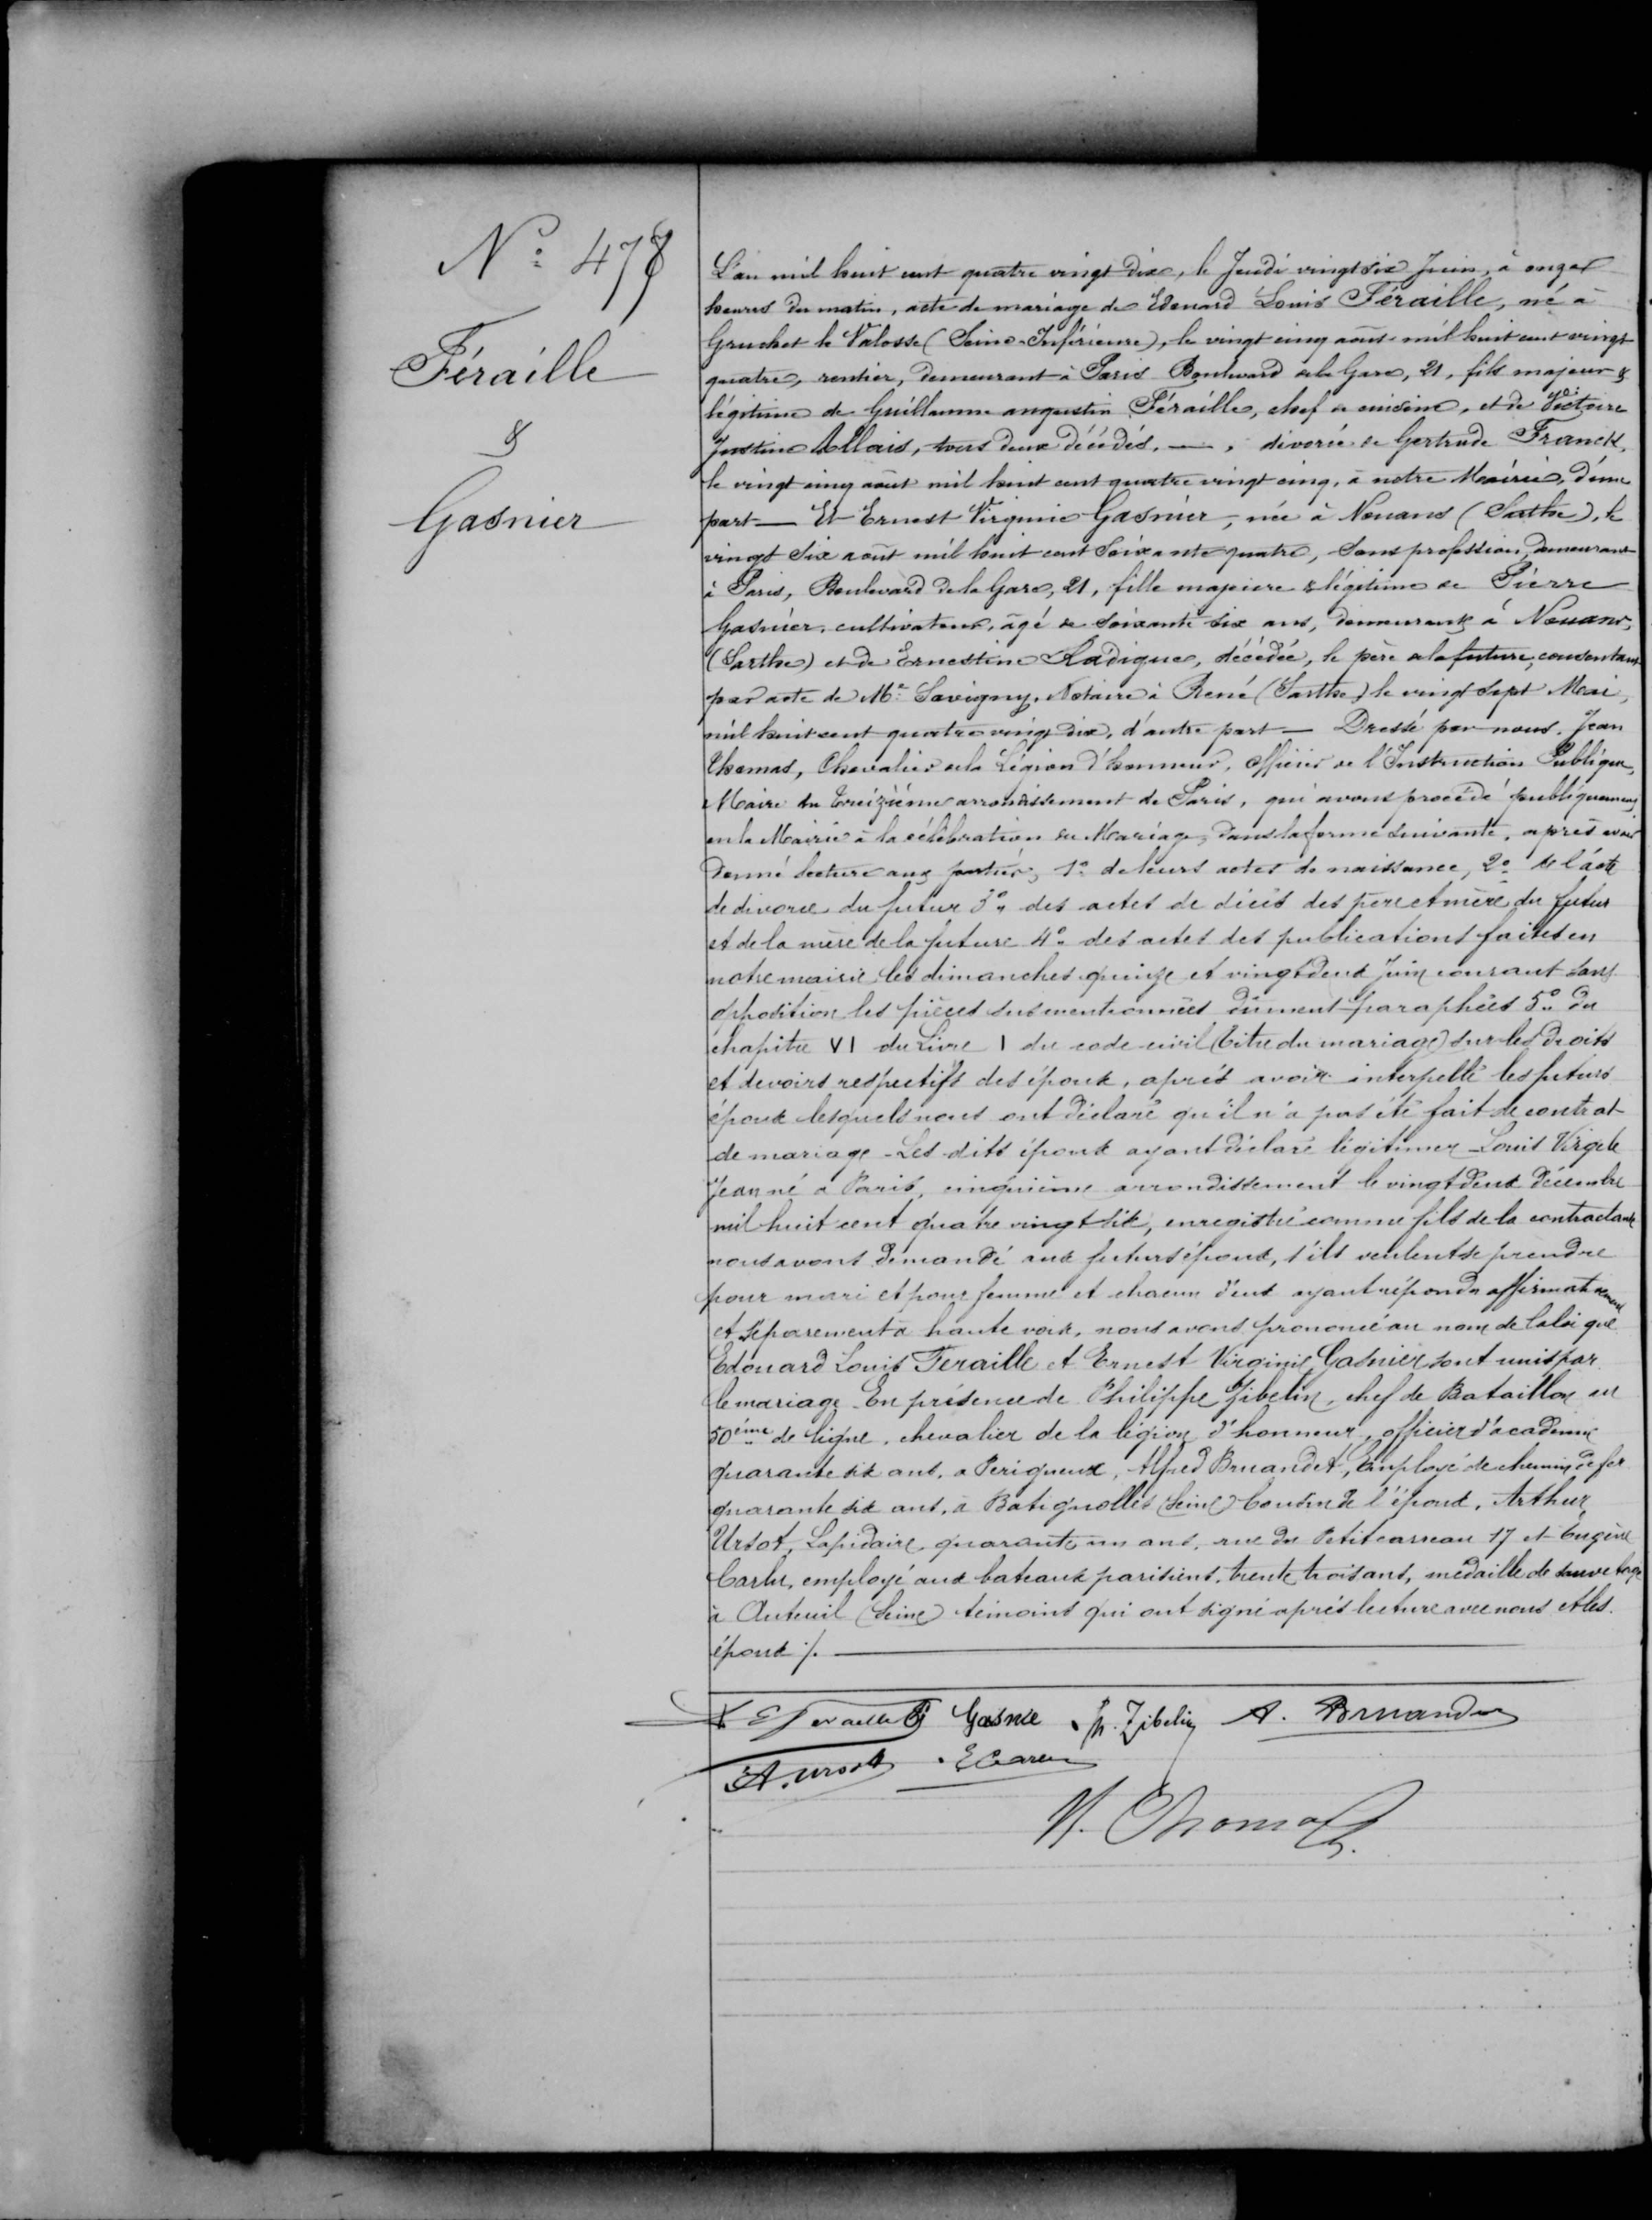N: 478
Féraille
&
Gasnier
L'an mil huit cent quatre vingt dix, le Jeudi vingt six Juin, à onze
heures du matin, acte de mariage de Edouard Louis Féraille, né à
Gruchet le Valosse (Seine Inférieure), le vingt-cinq août mil huit cent vingt
quatre, rentier, demeurant à Paris Boulevard de la Gare, 21, fils majeur &
légitime de Guillaume augustin Férille, chef de cuisine, et de Victoire
Justine Dollais, tous deux décédés, mariés, divorcé de Gertrude Franck,
le vingt-cinq août mil huit cent quatre vingt cinq, à notre Mairie, d'une
part.-Et Ernest Virginie Gasnier, née à Nouand (Sarthe), le
vingt-six août mil huit cent soixante quatre, sans profession, demeurant
à Paris, Boulevard de la Gare, 21, fille majeure & légitime de Pierre
Gasnier, cultivateur, âgé de soixante six ans, demeurant à Nouant,
(Sarthe) et de Erneestine Radiques, décédée, le père de le future, consentant
par acte de M°: Savigny, notaire à René (Sarthe) le vingt-sept Mai,
mil huit cent quatre vingt-six, d'autre part -Dressé par nous, Jean
Thomas, Chavlier de la Légion d'honneur, officier de l'instruction Publique,
Maire du treizième arrondissement de Paris, qu'avons procédé publiquement
en la Mairie à la célébration du Mariage dans la forme suivante, après avoir
donné lecture aux futurs, 1°/ de leurs actes de naissance, 2°/ de l'acte
de divorce du futur 3°/ des actes de décès des père et mère du futur
et de la mère de la future 4°/ des actes des publications faites en
notre mairie les dimanches quinze et vingt deux Juin courant sans
opposition les pièces susmentionnées dûment paraphées 5°/ du
chapitre VI du Livre I du code civil (titre du mariage) sur les droits
et devoirs respectifs des époux, après avoir interpellé les futurs
époux lesquels nous ont déclaré qu'il n'a pas été fait de contrat
de mariage. Les dits époux ayant déclaré légitimer Louis Virgile
Jeanne à Paris, cinquième arrondissement le vingt deux décembre
mil huit cent quatre vingt six, enregistré comme fils de la contractante
nous avons demandé aux futurs époux, s'ils veulent se prendre
pour mari et pour femme et chacun d'eux ayant répondu affirmativement
et séparement à haute voix, nous avons prononcé au nom de la loi que
Edouard Louis Féraille et Ernest Virginie Gasnier sont unis par
le mariage. En présence de Philippe Zibelin, chef de bataiillon au
50eme de ligne, chevalier de la légion d'honneur, officier d'académie
quarante-six ans, a Perigueux, Alfred Buandet, employé de chemin de fer
quarante six ans, à Batignolles (Seine) cousin de l'époux, Arthur
Ursot, Lapidaire, quarante un ans, rue du Petite carreau 17 et Eugène
Tarbu, employé aux bateaux parisiens, trente trois ans, medaille de sauvetage
à Auteuil (Seine) témoins qui ont signé après lecture avec nous et les
époux ./.
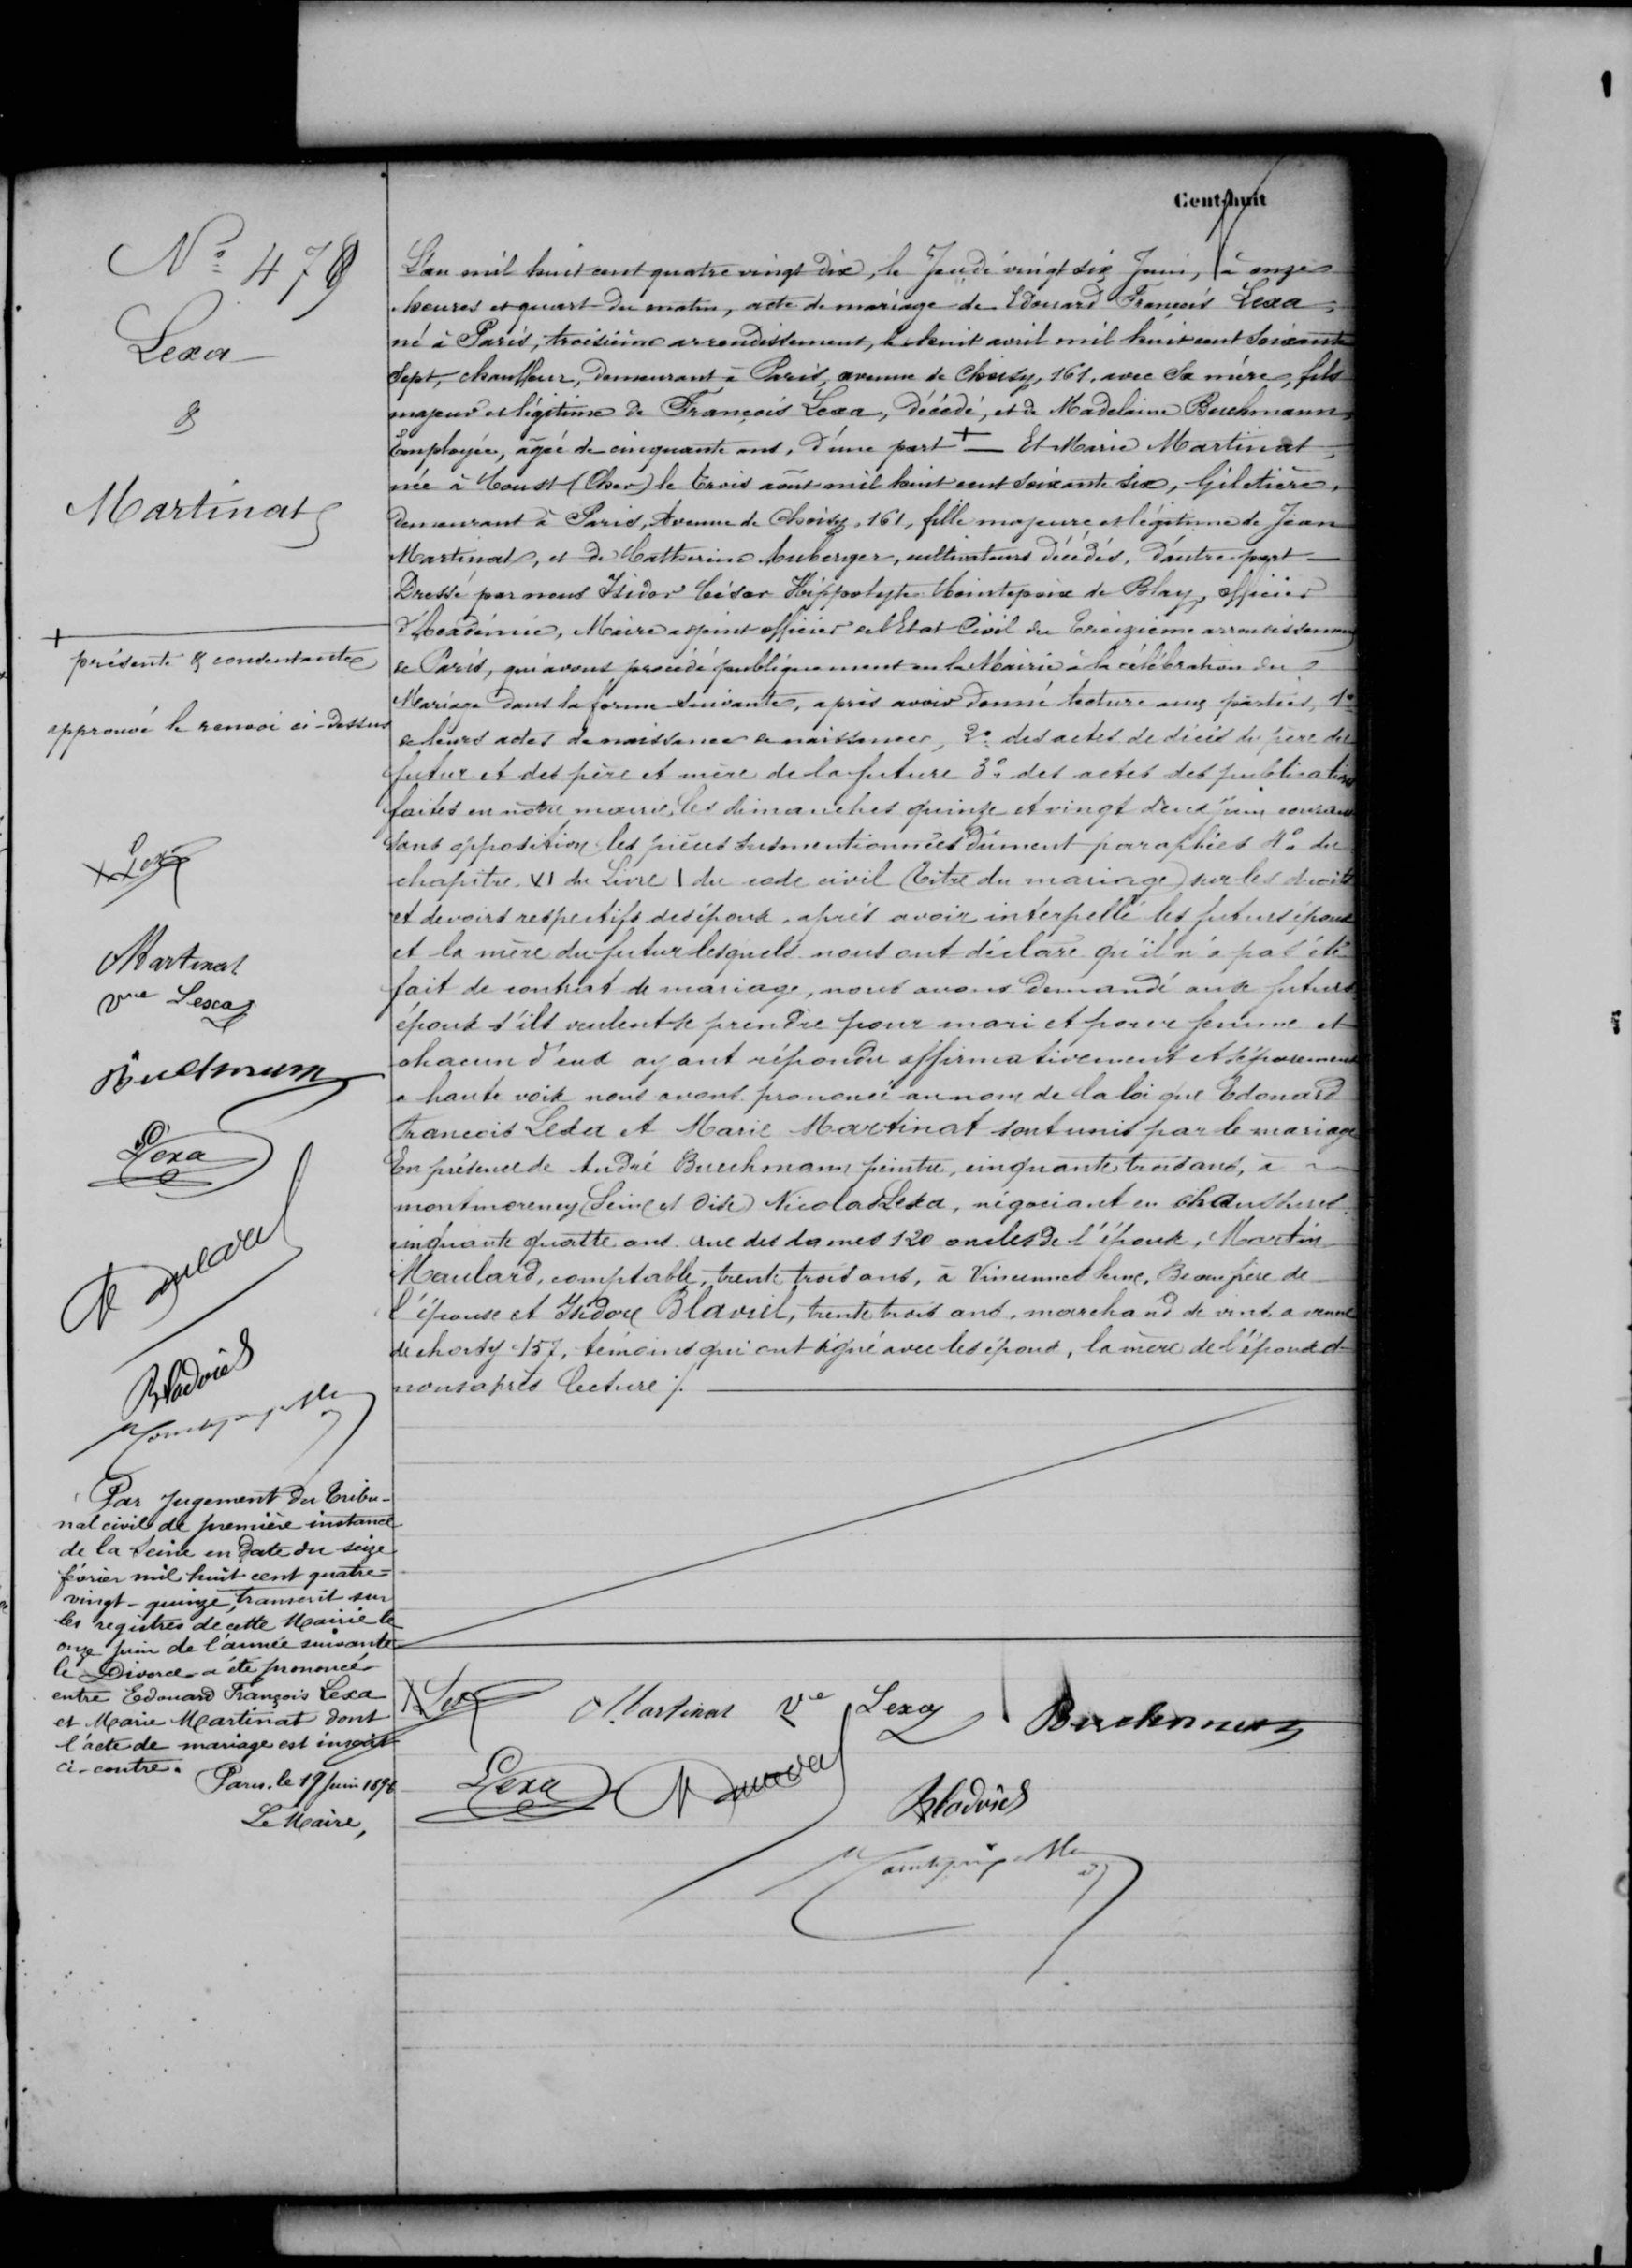N: 479
Lexa
&
Martinat
L'an mil huit cent quatre vingt dix, le Jeudi vingt six Juin à onzes
heures et quart du matin, acte de mariage de Edoard Francis Lexa,
né à Paris, troisième arrondissement, le huit avril mil huit cent soixante
sept, chauffeur, demeurant à Paris, avenue de Choissiy, 161, avec sa mère, fille
majeure et légitime de François Lexa, décédé et de Madeline Buchmann
Employée, âgée de cinquante ans, d'une part -Et Marie Martinat,
né à Noust (Cher) le trois août mil huit cent soixante six, Gilotière,
demeurant à Paris, Avenue de Choissy, 161, fille majeure et légitime de Jean
Martinat, et de Catherine Auberger, cultivateurs décédés, d'autre part -
Dressé par nous Isidor César Hippolyte Maintepaix de Bilay, officier
d'Académie, Maire adjoint officier de l'Etat Civil du treizième arrondissement
de Paris, qui avons procédé publiquement en la Mairie à la célébration du
Mariage dans la forme suivante, après avoir donné lecture aux parties, 1°:
de leurs actes de naissance de naissance, 2° des actes de décès du père du
futur et des père et mère de la future 3° des actes des publications
faites en notre mairie, les dimanches quinze et vingt deux Juin courant
sans opposition les pièces susmentionnées dûment paraphées 4°: du
chapitre VI du Livre I du code civil (Titre du mariage) sur les droits
et devoirs respectifs des époux, après avoir interpellé les futurs époux
et la mère du futur lesquels nous ont déclaré qu'il n'a pas été
fait de contrat de mariage, nous avons demandé aux futurs
époux s'ils veulent se prendre pour mari et pour femme et
chacun d'eux ayant répondu affirmativement et séparement
a haute voix nous avons prononcé au nom de la loi que Edouard
François Lexa et Marie Martinat sont unis par le mariage
En présence de André Buchmann peintre, cinquante trois ans, à
montmorency (Seine et Oise) Nicolas Lexa, négociant en chaussures
cinquante quatre ans rue des lames 120 oncles de l'époux, Martin
Mauclard, comptable, trente trois ans, à Vincennes Seine, Beau père de
l'épouse et Isidore Blaviel, trente tois ans, marchand de vins avenue
de choissy 157, témoins qui ont signé avec les époux, la mère de l'époux et
nous après lecture ./.